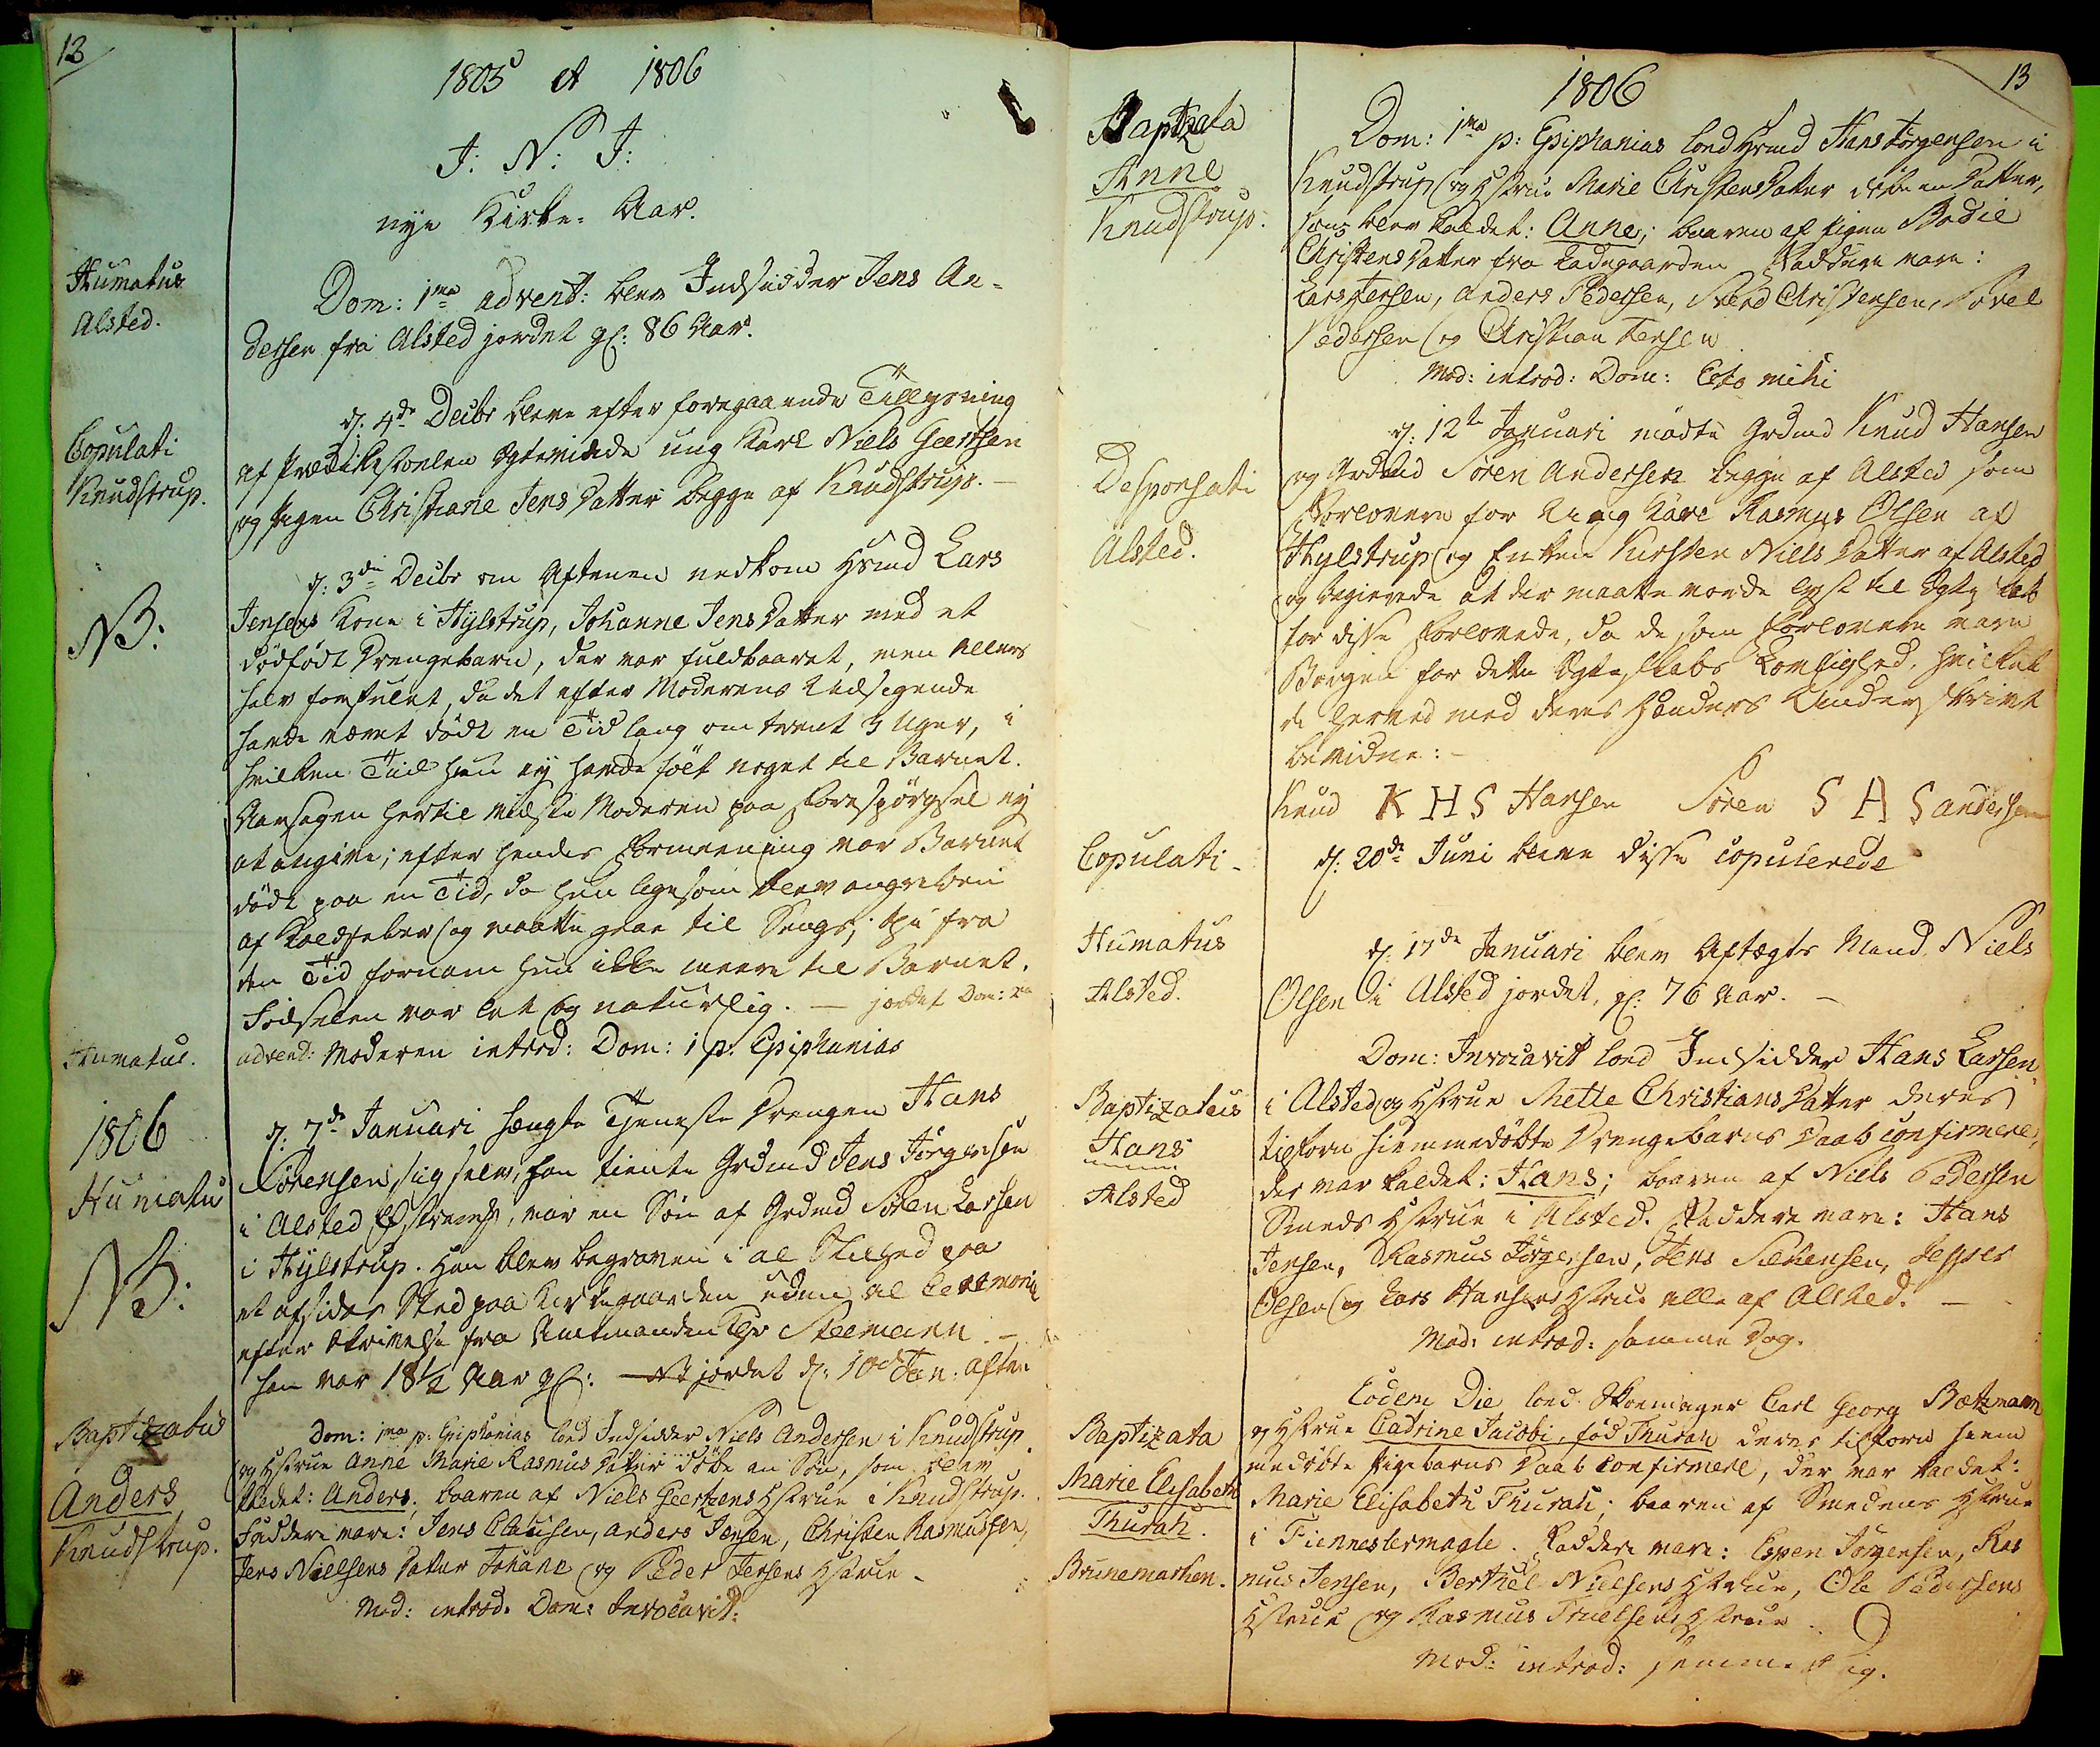12
Humatus
Alsted.
Copulati
Knudstrup.
NB.
Humatus.
1806
Humatus
NB:
Baptizatus
Anders
Knudstrup.
1805 et 1806
I: N: J:
nye Kirke = Aar.
Dom: 1ma Advent: blev Indsidder Jens An¬
dersen fra Alsted jordet gl: 86 Aar.
d. 4de Decbr bleve efter foregaaende Tillysning
af Prædikestolen ægteviede ung Karl Niels Geertsen
og Pigen Christiane Jens Datter begge af Knudstrup.
d: 3die Decbr om aftenen nedkom Hsmd Lars
Jensens Kone i Hylstrup, Johanne Jens Datter med et
dødfødt Drengebarn, der var fuldbaaret, men allers
halv forstulet, da det efter Moderens Udsigende
hande været dødt en Tid lang om trent 3 Uger, i
hvilken Tid, hun ey havde følt noget til Barnet.
Aarsagen hertil vidste Moderen paa Forespørgsel ey
at angive; efter hendes Formenning var Barnet
dødt paa en Tid, da hun ligesom blev angreben
af Koldfeber og maatte gaae til Sengs, ## fra
den Tid fornam hun ikke meere til Barnet.
Fødselen var let og naturlig: - jordet Dom: 2da
Advent: Moderen introd: Dom: 1 p. Epiphanias
d: 7de Januari Hængte Tjeneste Drengen Hans
Sørensen sig selv, han tiente Grdmd Jens Jørgensen
i Alsted Østrem##, var en Søn af Grdmd Sørren Larsen
i Hylstrup. Han blev begraven i al Stilhed paa
et afsides Sted paa Kirkegaarden uden al Ceremonie
efter Skrivelse fra Amtmanden Hlr Seemann.
han var 18½ Aar gl: ## jordet d: 10de  Jan: aften
Dom: 1ma p: Epiphanias loed Indsidder Niels Andersen i Knudstrup
og Hstrue Anne Marie Rasmus Datter døbe en Søn, som blev
kaldet Anders; baaren af Niels Geertzens Hstrue i Knudstrup.
Faddere vare: Jens Clausen, Anders Jensen, Christen Rasmussen
Jens Nielsens Datter Johanne og Peder Jensens Hstrue
Mod: introd: Dom: Invocavit:
Baptizata
Anne
Knudstrup.
Desponsati
Alsted.
Copulati
Humatus
Alsted.
Baptizatus.
Hans
Alsted.
Baptizata
Marie Elisabeth
Thurah
Brunemarken.
13
1806
Dom: 1ma p: Epiphanias loed Hsmd Hans Jørgensen i
Knudstrup og Hstrue Marie Christens Datter døbe en Datter
som blev kaldet: Anne; baaren af Pigen Bodil
Christens Datter fra Ladegaarden. Faddere vare:
Lars Jensen, Anders Pedersen, Svend Christensen, Povel
Pedersen og Christian Jensen
Mod: introd: Dom: Esto mihi
d: 12te Januari mødte Grdmd Knud Hansen
og Grdmd Søren Andersen begge af Alsted som
Forlovere for Ung Karl Rasmus Olsen af
Hylstrup og Enken Kirsten Niels Datter af Alsted
og begierede at der maatte vorde lyst til Ægteskab
for disse Forlovede, da de som forlovere vare
Borgen for dette Ægteskabs Lovlighed, hvilket
de herved med deres Hænders Underskrivt
bevidne:
Knud K H S Hansen Søren  S A S Andersen
d: 20de Juni bleve disse copulerede
d: 17de Januari blev Aftægts Mand, Niels
Olsen i Alsted jordet gl: 76 Aar.
Dom: Invocavit loed Indsidder Hans Larsen
i Alsted og Hstrue Mette Christians Datter deres
tilforn hiemmedøbte Drengebarns Daab confirmere
der var kalde:t Hans; baaren af Niels Pedersen
Smeds Hstrue i Alsted. Faddere vare: Hans
Jensen, Rasmus Jørgensen, Jens Silkensen, Jesper
Olsen og Lars Hansens Hstrue alle af Alsted.
Mod: introd: samme Dag.
Eodem Die loed Skoendager, Carl Georg Bætzmann
og Hstrue Catrine Jacobi, fød Thurah deres tilforn hiem
medøbte Pigebarns Daab confirmere, der var kaldet:
Marie Elisabeth Thurah; baaren af Smedens Hstrue
i Fienneslevmagle. Faddere vare: Espen Jørgensen, Ras
mus Jensen, Berthel Nielsens Hstrue, Ole Pedersens
Hstrue og Rasmus Truelsens Hstrue
Mod: introd: sammed Dag.
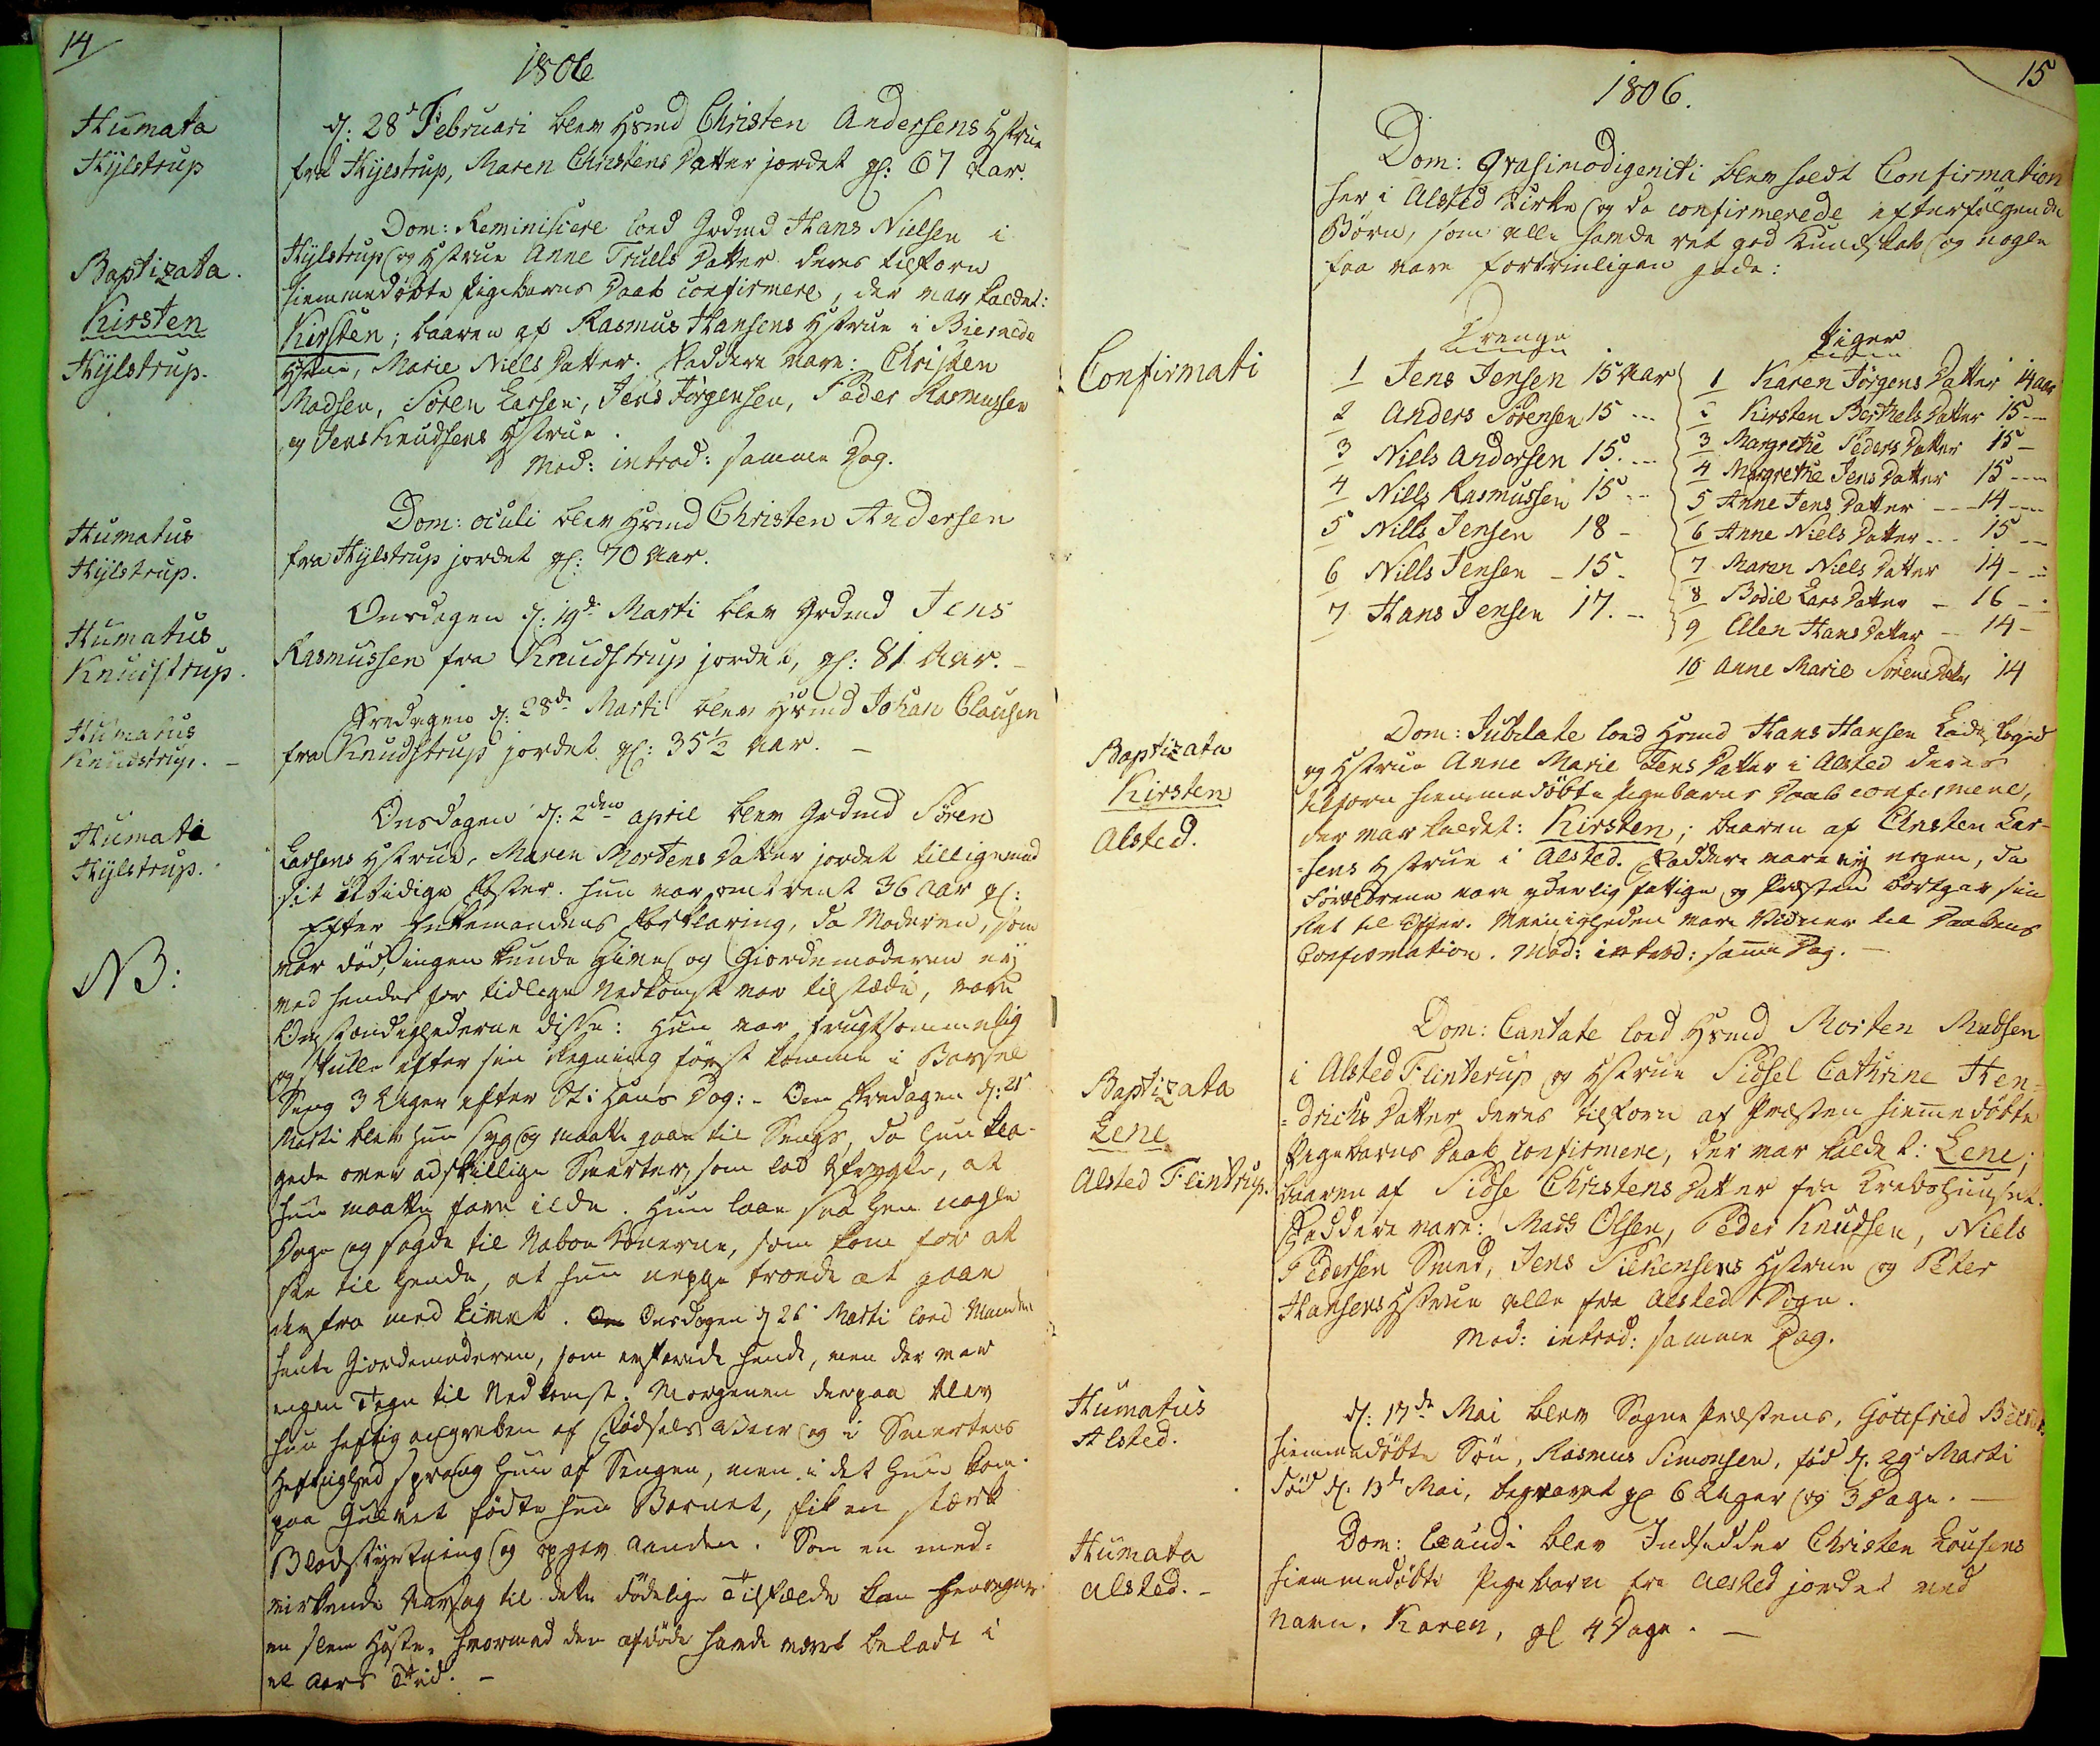14
Humata
Hylstrup
Baptizata.
Kirsten
Hylstrup.
Humatus
Hylstrup.
Humatus
Knudstrup.
Humatus
Knudstrup.
Humati
Hylstrup.
NB;
1806
d: 28 Februari blev Hsmd Christen Andersens Hstrue
fra Hylstrup, Maren Christens Datter jordet gl: 67 Aar.
Dom. Reminisiere loed Grdmd Hans Nielsen i
Hylstrup, og Hstrue Anne Truels Datter deres tilforn
hiemmedøbte Pigebarns Daab confirmere, der var kaldet:
Kirsten; baaren af Rasmus Hansens Hstrue i Biernede
Hsene##, Marie Niels Datter. Faddere vare: Christen
Madsen, Søren Larsen, Jens Jørgensen, Peder Rasmussen
og Jens Knudsens Hustrue
Mod: introd: samme Dag.
Dom: oculi blev Hsmd Christen Andersen
fra Hylstrup jordet gl: 70 Aar.
Onsdagen d: 19de Marti blev Grdmd Jens
Rasmussen fra Knudstrup jordet gl: 81 Aar.
Fredagen d: 28de Marti blev Hsmd Johan Clausen
fra Knudstrup jordet gl: 35½ Aar.
Onsdagen d: 2den April blev Grdmd Søren
Larsens Hstrue, Maren Mortens Datter jordet tilligemed
sit ##tidige Foster. hun var omtrent 36 Aar gl:
Efter Enkemandens Forklaring, da Moderen, som
var død, ingen kunde give og Giordemoderen ey
ved hendes for tillige nedkomst var tilstæde, vare
Omstændighederne disse: Hun var frugtsommelig
og skulle efter sin Regning først komme i Barsel
Seng 3 Uger efter St: Hans Dag: - Om Fredagen d: 21##
Marti blev hun syg og maatte gaae til Sengs, da hun kla¬
gede over adskillige Smerter, som lod ##feygste, at
hun maatte fare ilde. Hun laae saa hen nogle
Dage og sagde til Naboe Konerne, som kom for at
see til hende, at hun neppe troede at gaae
der fra med Livet. Om Onsdagen d 26 Marti loed Manden
hente Giordemoderen, som ##de hende, men der var
ingen Tegn til Nedkomst. Morgenen derpaa blev
hun heftig angreben af Fødsels Veer i Smertens
Heftighed sprang hun af Sengen, men i det hun kom
paa Gulvet fødte hun Barnet, fik en stærk
Blodstyrtning og opgav Aanden. Som en med¬
virkende Aarsag til dette dødelige Tilfælde kan ##
en slem Hoste, hvormed den afdøde havde været bela## i
et Aars Tid.
Confirmati
Baptizata
Kirsten
Alsted.
Baptizata
Lene
Alsted Flinterup.
Humatus
Alsted.
Humata
Alsted.
15
1806
Dom: Qvasimodigeniti blev holdt Confirmation
her i Alsted Kirke og da confirmerede efterfølgende
Børn, som alle havde ret god Knudskab og nogle
faa vare fortrinligen gode:
Drenge
1 Jens Jensen 15 Aar
2 Anders Sørensen 5 ---
3 Niels Andersen 15 ---
4 Niels Rasmussen 15 ---
5 Niels Jensen 18 -
6 Niels Jensen - 15 -
7 Hans Jensen 17. --
Piger
1 Karen Jørgens Datter 14 Aar
2 Kirsten Berthels Datter 15 ---
3 Margrete Peders Datter 15 -
4 Margrete Jens Datter 15 ----
5 Anne Jens Datter ---14 ----
6 Anne Niels Datter ---15 ---
7 Maren Niels Datter 14.
8 Bodil Lars Datter - 16 -
9 Ellen Hans Datter -- 14 -
10 Anne Marie Sørens Datter 14
Dom: Jubilate loed Hsmd Hans Hansen Ladefoged
og Hstrue Anne Marie Jens Datter i Alsted deres
tilforn hiemmedøbte Pigebarns Daab confirmere,
der var kaldet: Kirsten; baaren af Christen Lar¬
= sens Hustrue i Alsted. Faddere vare ey nogen, da
Forældrene vare ydenlig fattige og Præsten bortgav sin
Ret til Offer. Meenigheden vare Vidner til Daabens
Confirmation. Mod: introd: samme Dag.
Dom: Cantate loed Hsmd Morten Madsen
i Alsted Flinterup og Hstrue Sidsel Cathrine Hen¬
drichs Datter deres tilforn af Præsten hiemmedøbte
Pigebarns Daab confirmere, der var kaldet: Lene;
baaren af Sidse Christens Datter fra Krebohuuset.
Faddere vare: Mads Olsen, Peder Knudsen, Niels
Pedersen Smed, Jens Silkensens Hstrue og Peter
Hansens Hstrue alle fra Alsted Sogn.
Mod: introd: samme Dag.
d: 17de Mai blev Sogne Præstens Godttfried Becker##
hiemmedøbte Søn, Rasmus Simonsen, fød d. 29de Marti
død d. 13de Mai begravet gl 6 Uager og 3 Dage.
Dom: Exaudi blev Indsidder Christen Lausens
hiemmedøbte Pigebarn fra Alsted jordet ved
Navn Karen, gl 4 Dage.